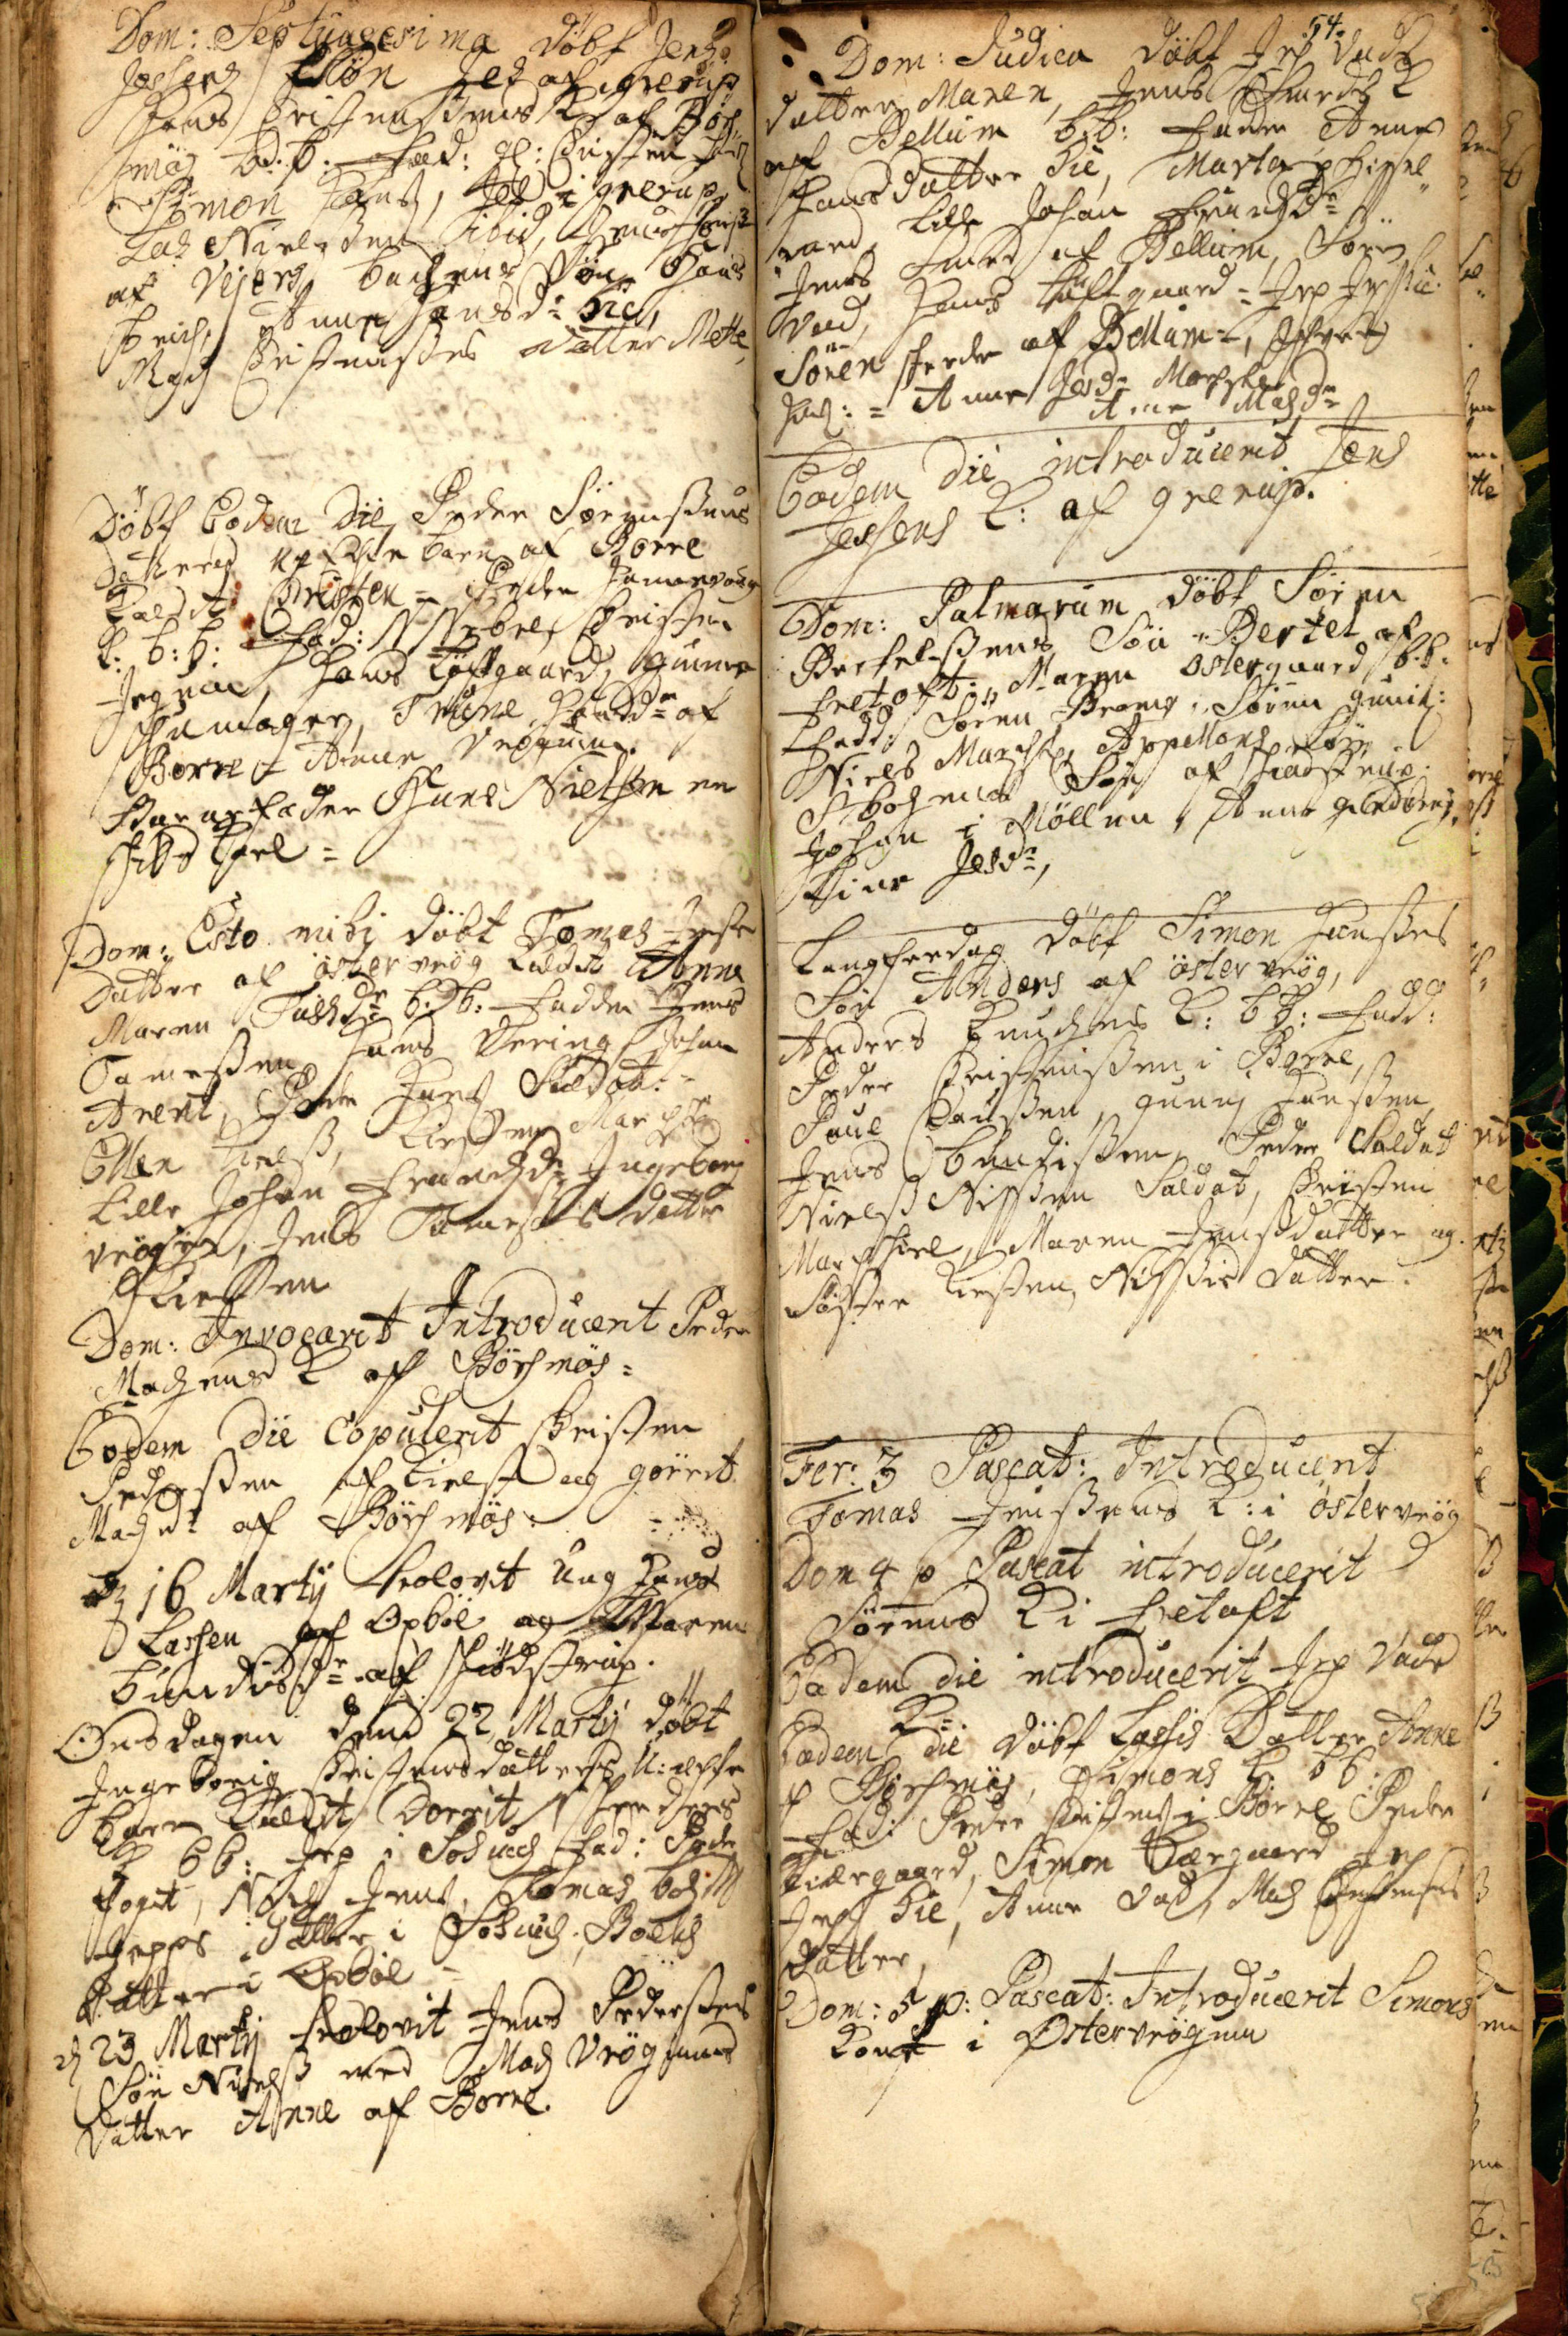Dom: Septuagesima døbt Jens
Jessens Søn Jes af Grerup
Hans Christensens K af Børs¬
møs b:b: Fad: gl. Christen Hans
Simon Hans, Jes i Grerup
Las Nielsen ibid, Jens Jonsen
af Vejers, Bachens Søn Hans
Brich, Anne Hansdr hic##,
Madz Christensens Datter Mette
Døbt Eodem Die Peder Sørensens
Datters uæchte Barn af Borre
kaldit Christen= Peder Hannevangs
K: b:b: Fad: N Nebel, Christen
Jegum, Hans Løftgaard, Gunne
Schumager, Trune Hansdr af
Borre= Anne Vrøgum.
Barnefader Hans Nielsen en
Schibs Karl=
Dom: Esto mihi døbt Tomas Jens
Datter af Østervrøg kaldit Anne
Maren Tuesdr b:b: Faddere Jens
Tomesen Hans Vering, Johan
Arent, Peder Hans Soldat:
Ellen Kiels, Kirsten Marchsch
Lille Johan Frandzdr Ingeborg
Vrøgum, Jens Tomesens Datter
Kirsten
Dom. Invocavit Introducerit Peder
Madzens K af Børsmos=
Eodem Die Copulerit Christen
Pedersen af Kielst og Gørrit
Madzdr af Børsmøs:
D 16 Martij trolovet Ung Hans
Lassen af Oxbøl og Maren
Bundisdr af Schiødstrup.
Onsdagen dend 22 Martij døbt
Ingeborig Christensdatters U:æchte
Barn kaldit Dorrit, N Schreders
K bb: Jep i Søhuus Fad: Peder
Fogit, Niels Jens, Tomas Bodz M##
Jeppes Datter i Søhuus, Bol##s
Datter i Oxbøl=
D 23 Martij trolovit= Jens Pedersens
Søn Niels med Madz Vrøgums
Datter Anne af Borre.
54.
Dom: Judica døbt Jep Vads
Datter Maren, Jens Smeds K
af Bellum b:b: Faddere Anne
Hansdatter hic##, Marta p Hissel¬
med, Lille Johan Frandzdr
Jens Smed af Bellum, Søren
Vad, Hans Løftgaard= Jep Je##.
Søren Schreder af Bellum=, Ifver
Hans:= Anne Jesdr Marchs##
Anne Madzdr
Eodem Die introducerit Jens
Jessens K: af Grerup.
Dom:Palmarum døbt Søren
Bertelsens Søn .,. Bertel af
Fretoft: Maren Østergaard b.b.
Fadd: Søren Broeng, Søren Gunis:
Niels Marchs, Appellons Søn.
S. Bodzens Søn af Schiødstrup.
Johan i Møllen, Anne Giedbrig,
Line Jesdr,
Langfredag døbt Simon Hansens
Søn Anders af Østervrøg,
Anders Knudzens K: bb: Fadd:
Peder Christensen i Borre,
Poul Clausen, Gunnj Hansen
Jens Bundisen, Peder Soldat
Niels Nissen Soldat, Christen
Marchschiel, Maren Jensdatter og
Søster, Kirsten Nissis Datter.
Fer: 3 Pascat: introducerit
Tomas Jensens K: i Østervrøg
Dom 4 p Pascat introduceret
Sørens K i Fretoft
Eodem Die introducerit Jep Vads
K=.
Eodem Die døbt Lassis Datter Anne
af Børsmøs, Simons K: bb:
Fad: Peder Christens i Borre Peder
Kiærgaard, Simon Kiærgaard, Jep
Jepsen hic##, Anne Vad, Madz Christensens
Datter.
Dom: 5 p: Pascat: Introducerit Simons
Kone i Østervrøgum
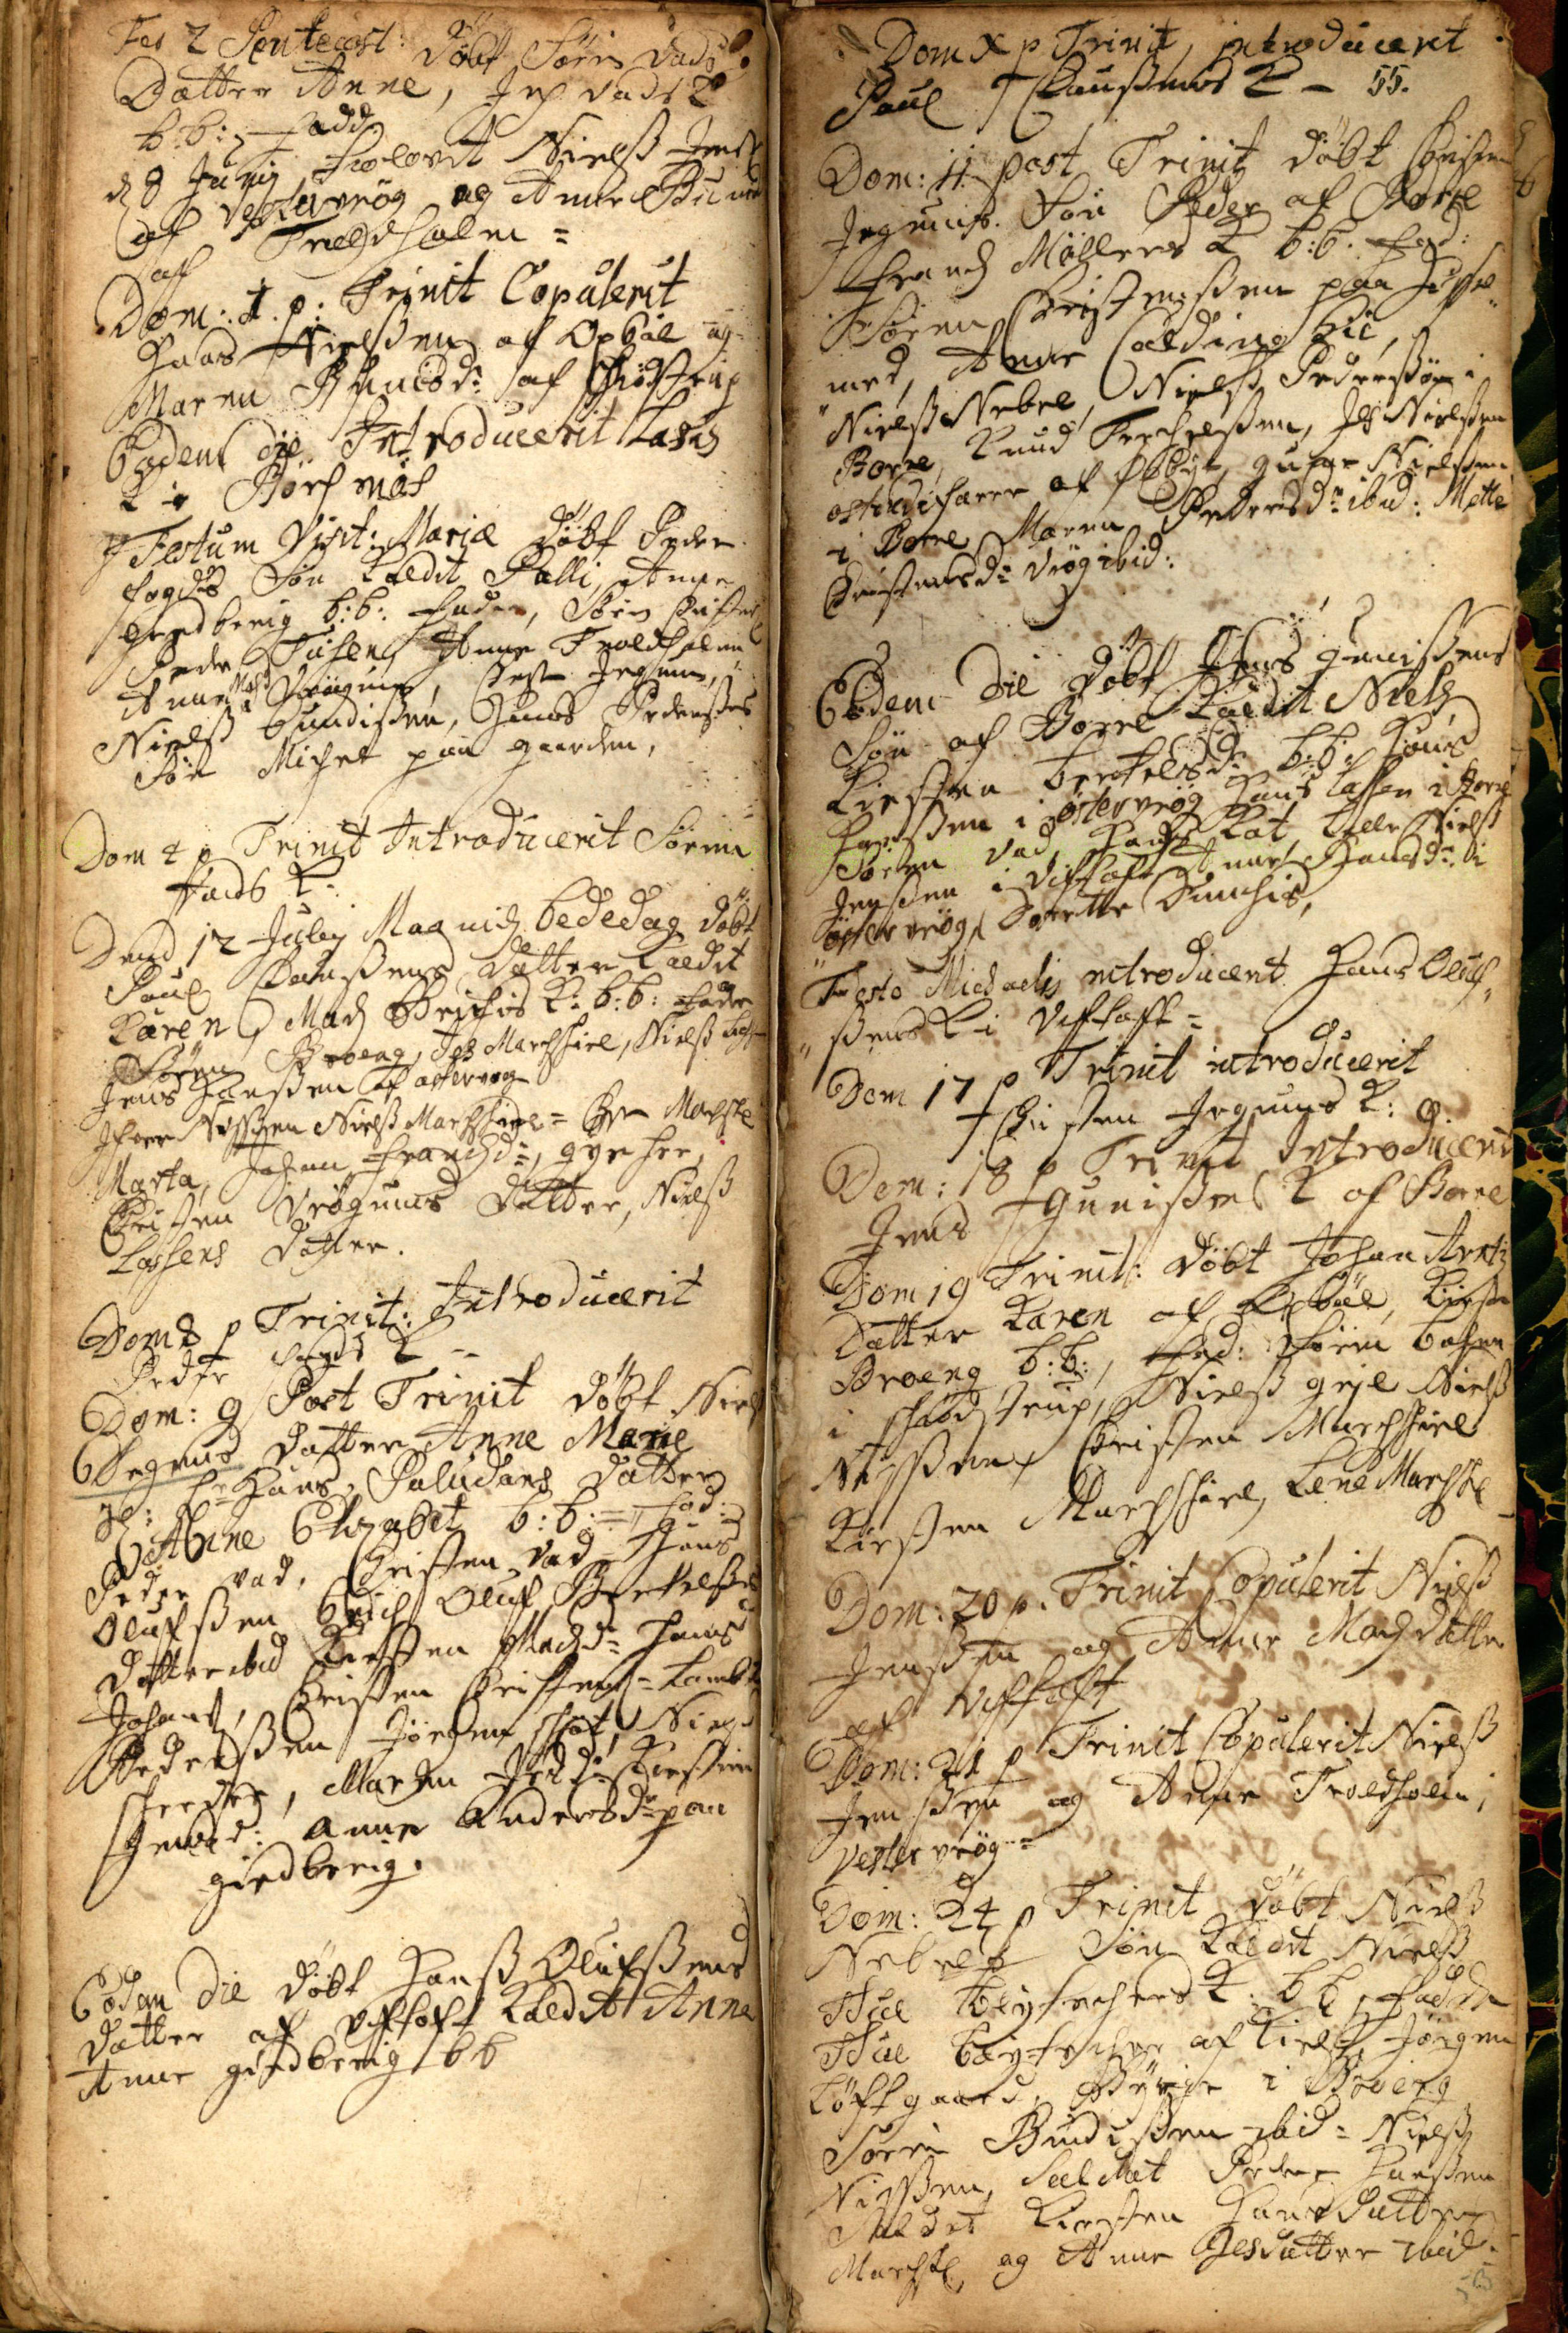Fer 2 Pentecost: døbt Søren Vads
Datter Anne, Jep Vads K
b:b: fadd:
D 8 Junij trolovit Niels Jens
af Vestervrøg og Anne Bunid##
af Troldholm=
Dom: 1. p:Trinit Copulerit
Hans Nielsen af Oxbøl og
Maren Bunisdr af Schiødstrup
Eodem Die Introducerit Lasis
K i Børsmøs
Festum Visit: Mariæ døbt Peder
Fogdis Søn kaldit Palli, Anne
Giedberig b:b: Fadre, Søren Christens
Peder Tusen, Anne Troldholm
Anne Madzd Vrøgum, Christen Jegum,
Niels Bundisen, Hans Pedersens
Søn Michel paa Gaarden,
Dom 2 p Trinit Introducerit Søren
Vads K.
Dend 12 Julij Maanedz Bededag døbt
Poul Clausens Datter kaldit
Karen, Madz Brichis K: b:b: Fadr
Søren Broeng, Jes Marchschiel, Niels Bo##
Jens Hansen af Østervrøg
Ifver Nissen Niels Marchschiel= Chr Marchsch
Marta, Johan Frandzdr, Gye## her
Christen Vrøgums Datter, Niels
Lassens Datter
Dom 8 p Trinit: Introducerit
Peder Fogdis K=
Dom: 9 Post Trinit døbt Niels
Degens Datter Anne Marie
Sl: ##r Hans Paludans Datter
Anne Elizabet b:b: =,, Fad:
Peder Vad, Christen Vad, Jens
Olufs Brich Oluf Bertelsens
Datter ibid Kirsten Madzdr Hans
Johans, Christen Christens= Lamb##
Pedersen, Jørgen Schøt, Niels
Schreder, Maren Jesdr Kirsten
Jensd: Anne Andersdr paa
Giedberig.
Eodem Die døbt Hans Olufsens
Datter af Viftoft kaldit Anne,
Anne Giedberig bb
55
53
Dom X p Trinit Introducerit
Poul Clausens K-
Dom: 11. post Trinit døbt Christen
Jegums. Søn Peder af Borre
Frandz Møllers K b:b. Fad:
Søren Christensen paa Hissel¬
med, Anne Colding hic##,
Niels Nebel, Niels Pedersøn i
Borre, Knud Terchelsen, Jes Nielsen
Ostindifarer af Oxbye, Gunne Nielsen
i Borre Maren Pedersdr ibid: Mette
Christensdr i Vrøg ibid:
Eodem Die døbt Jens Gunisens
Søn af Borre kaldit Niels
Kirsten Bertelsdr b:b: Hans
Hansen i Østervrøg Hans Lassen i Borre,
Søren Vad, Hans Kot, Lille Niels
Jensen i Viftoft, Anne Hansdr i
Østervrøg, Dorrette D##is
Festo Michaelis introducerit Hans Oluf¬
sens K i Viftoft=
Dom.17 p Trinit introducerit
Christen Jegums K:
Dom: 18 p Trinit Introducerit
Jens Gunisens K af Borre
Dom 19 Trinit: døbt Johan Arntz
Datter Karen af Oxbøl, Kirsten
Broeng b:b: Fad: Søren Bosen
i Schiødstrup, Niels Gejl Niels
Nissen, Christen Marchschiel
Kirsten Marchschiel, Lene Marchsch
Dom:20 p. Trinit Copulerit Niels
Jensen og Anne Madzdatter
af Viftoft
Dom: 21 p Trinit Copulerit Niels
Jensen og Anne Troldholm i
Vestervrøg=
Dom: 24 p Trinit døbt Niels
Nebels Søn kaldit Niels
Thue Bertelsens K: bb Fadd
Thue Bertelsen## af Kielst, Jørgen
Løftgaard, Byrge i Broeng
Søren Bundisen ibid= Niels
Nissen Soldat Peder Hansen
Soldat, Kirsten Hansdatter
Marchs## og Anne Jesdatter ibid.